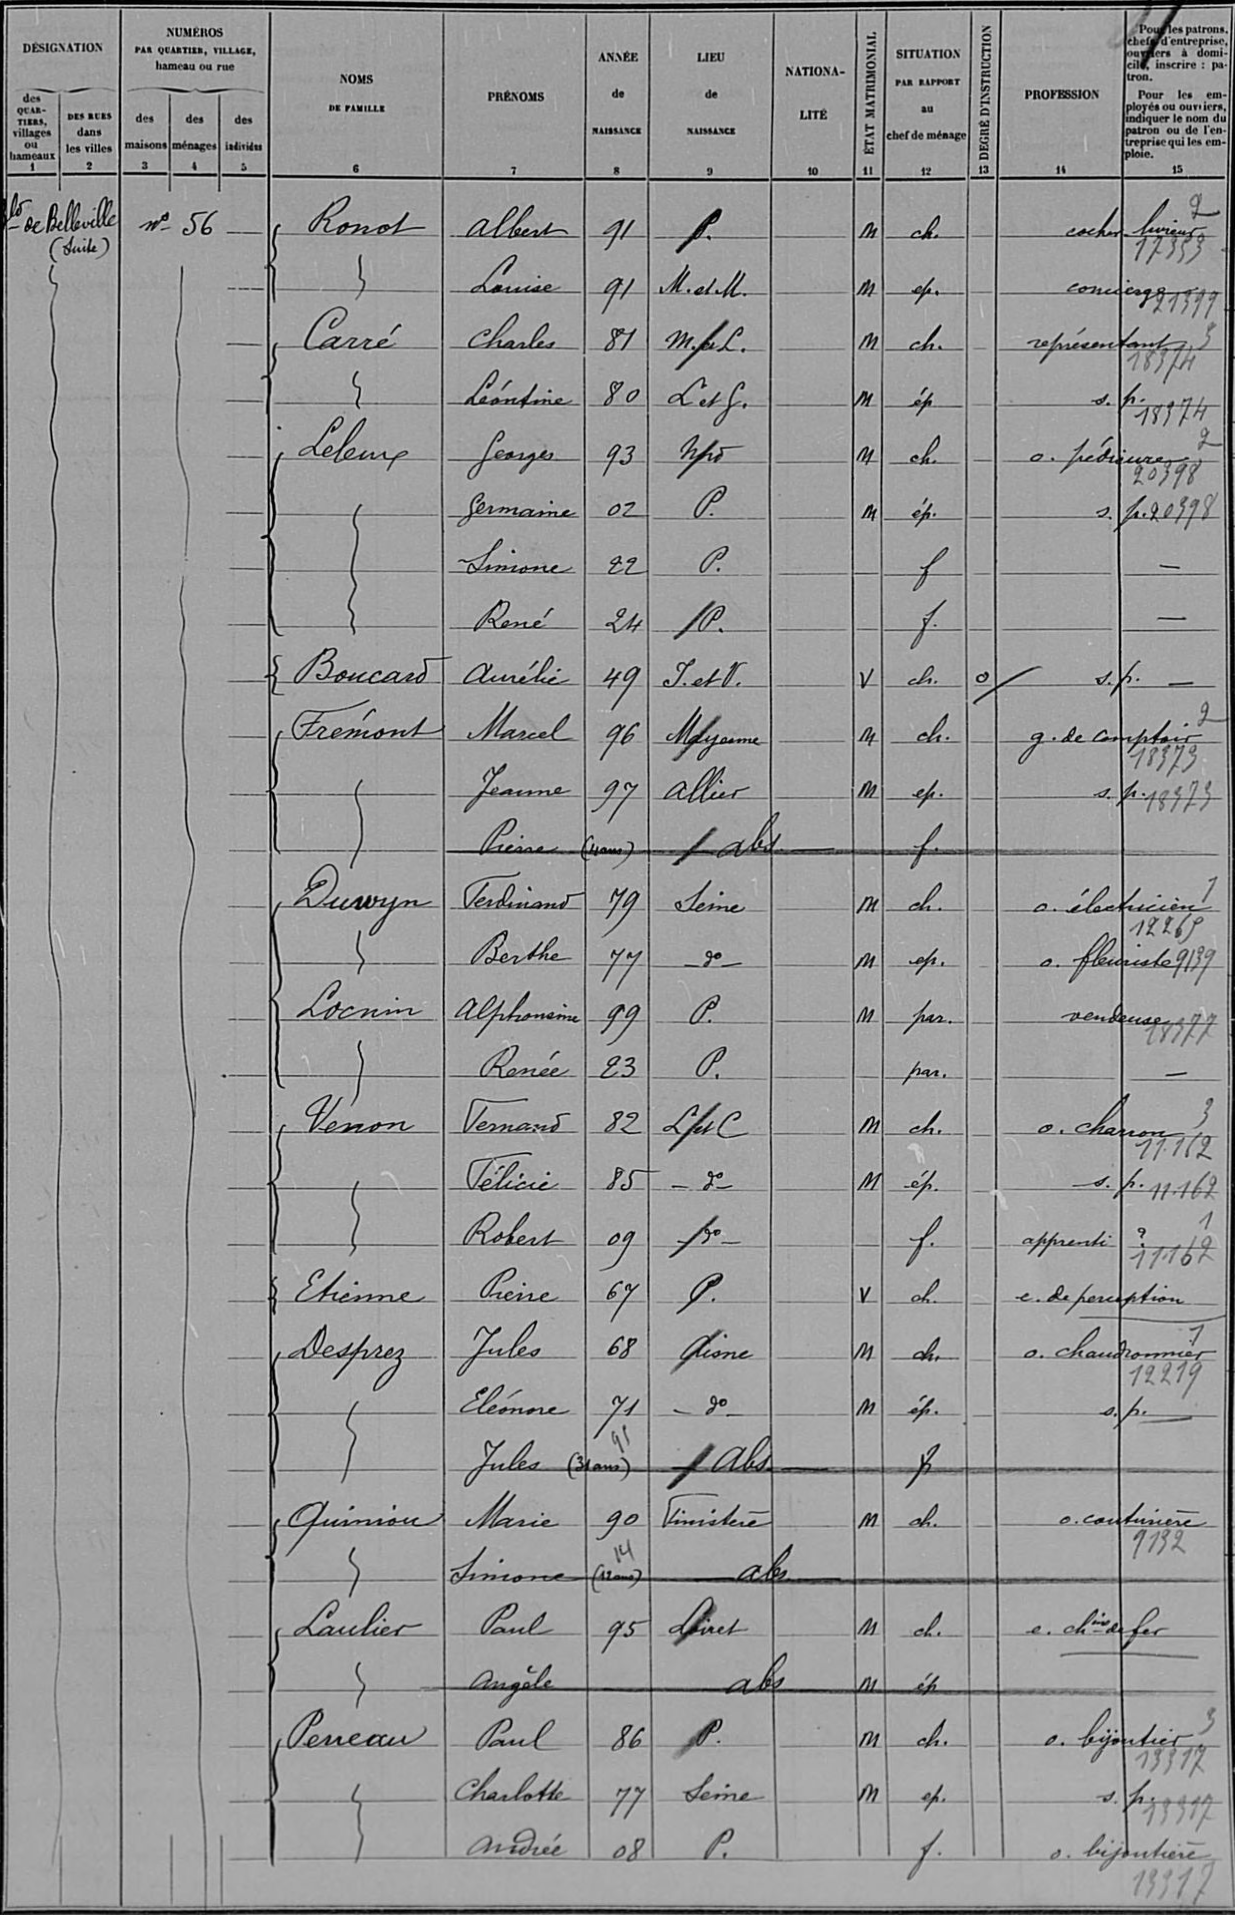Ronot/Albert/91/P /¤/M/ch /¤/cocher-livreur/¤
¤/Louise/91/M et M /¤/M/ep /¤/concierge/¤
Carré/Charles/81/M et L /¤/M/ch /¤/représentant/¤
¤/Léontine/80/L et G /¤/M/ép/¤/s p /18374
Leleux/Georges/93/Nord/¤/M/ch /¤/o pédicure/¤
¤/Germaine/02/P /¤/M/ép /¤/s p /20398
¤/Simone/22/P /¤/¤/f/¤/¤/¤/¤
¤/René/24/P /¤/¤/f /¤/¤/¤/¤
Boucard/Aurélie/49/I et V /¤/V/ch /o/s p /¤/¤
Frémont/Marcel/96/Mayenne/¤/M/ch /¤/g de comptoir/¤
¤/Jeanne/97/Allier/¤/M/ep /¤/s p /18373
¤/Pierre/(4 ans)/abs/¤/¤/f /¤/¤/¤/¤
Duwyn/Ferdinand/79/Seine/¤/M/ch /¤/o électricien/¤
¤/Berthe/77/d°/¤/M/ep /¤/o fleuriste/9139
Locnin/Alphonsine/99/P /¤/M/par /¤/vendeuse/¤
¤/Renée/23/P /¤/¤/par /¤/¤/¤/¤
Venon/Fernand/82/L et C/¤/M/ch /¤/o charron/11162 3
Félicie/85/d°/¤/M/ép /¤/s p /11162 1
¤/Robert/09/d°/¤/¤/f /¤/apprenti/¤
Etienne/Pierre/67/P /¤/V/ch /¤/e de perception/¤/¤
Desprez/Jules/68/Aisne/¤/M/ch /¤/o chaudronnier/¤
¤/Eléonore/71/d°/¤/M/ép /¤/s p /¤/¤
¤/Jules/(31 ans) 95/abs/¤/¤/f/¤/¤/¤
Quiniou/Marie/90/Finistère/¤/M/ch /¤/o couturière/¤
¤/Simone/(12 ans) 14/abs/¤/¤/¤/¤/¤/¤/¤
Laulier/Paul/95/Loiret/¤/M/ch /¤/e chin de fer/¤/¤
¤/Angèle/¤/abs/¤/M/ép/¤/¤/¤/¤
Perreau/Paul/86/P /¤/M/ch /¤/o bijoutier/¤
¤/Charlotte/77/Seine/¤/M/ep /¤/s p /13317
¤/Andrée/08/P /¤/¤/f /¤/o bijoutière/¤
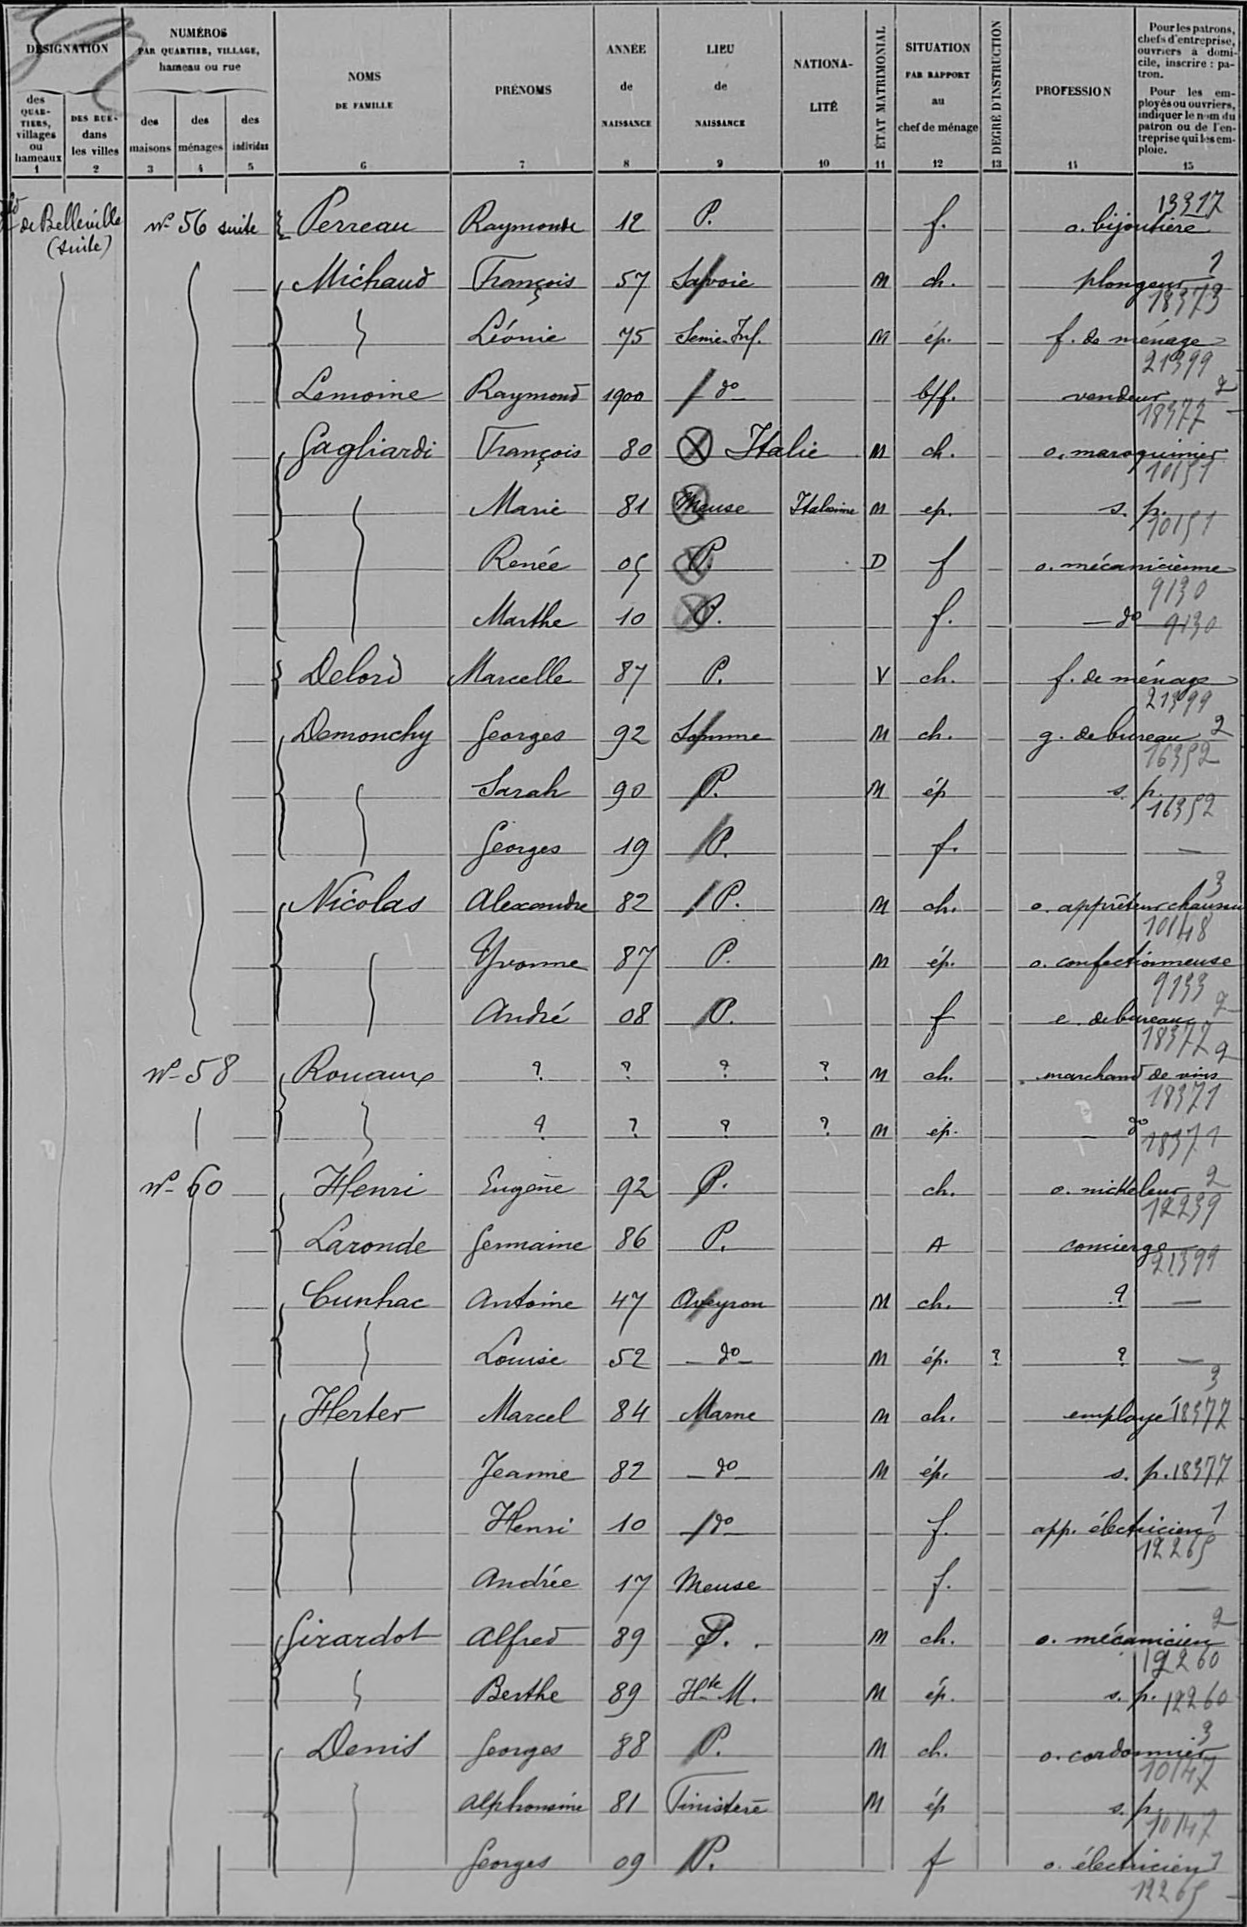Perreau/Raymonde/12/P /¤/¤/f /¤/o bijoutière/¤
Michaud/François/57/Savoie/¤/M/ch /¤/plongeur/¤
¤/Léonie/75/Seine Inf /¤/M/ép /¤/f de ménage/¤
Lemoine/Raymond/1900/d°/¤/¤/b-f /¤/vendeur/¤
Gagliardi/François/80/Italie/¤/M/ch /¤/o maroquinier/¤
¤/Marie/81/Meuse/Italienne/M/ep /¤/s p /¤
¤/Renée/05/P /¤/D/f/¤/o mécanicienne/¤
¤/Marthe/10/P /¤/¤/f /¤/d°/9130
Delord/Marcelle/87/P /¤/V/ch /¤/f de ménage/¤
Demonchy/Georges/92/Somme/¤/M/ch /¤/g de bureau/¤
¤/Sarah/90/P /¤/M/ép/¤/s p /¤
¤/Georges/19/P /¤/¤/f /¤/¤/¤/¤
Nicolas/Alexandre/82/P /¤/M/ch /¤/o apprêteur chausseur/¤
¤/Yvonne/87/P /¤/M/ép /¤/o confectionneuse/¤
¤/André/08/P /¤/¤/f/¤/e de bureaux/¤
Rouaux/¤/¤/¤/¤/M/ch /¤/marchand de vins/¤
¤/¤/¤/¤/¤/M:ép /¤/s°/18371
Henri/Eugène/92/P /¤/¤/ch /¤/o nickeleur/¤
Laronde/Germaine/86/P /¤/¤/A/¤/concierge/¤
Cunhac/Antoine/47/Aveyron/¤/M/ch /¤/¤/¤/¤
¤/Louise/52/d°/¤/M/ép /¤/¤/¤/¤
Herter/Marcel/84/Marne/¤/M/ch /¤/employé/18577
¤/Jeannie/82/d°/¤/M/ép /¤/s p /18377
¤/Henri/10/d°/¤/¤/f /¤/app électricien/¤
¤/Andrée/17/Meuse/¤/¤/f /¤/¤/¤
Girardot/Alfred/89/P /¤/M/ch /¤/o mécanicien/¤
¤/Berthe/89/Hte M /¤/M/ép /¤/s p /12260
Denis/Georges/88/P /¤/M/ch /¤/o cordonnier/¤
¤/Alphonsine/81/Finistère/¤/M/ép/¤/s p /10147
¤/Georges/09/P /¤/¤/f/¤/o electricien/¤
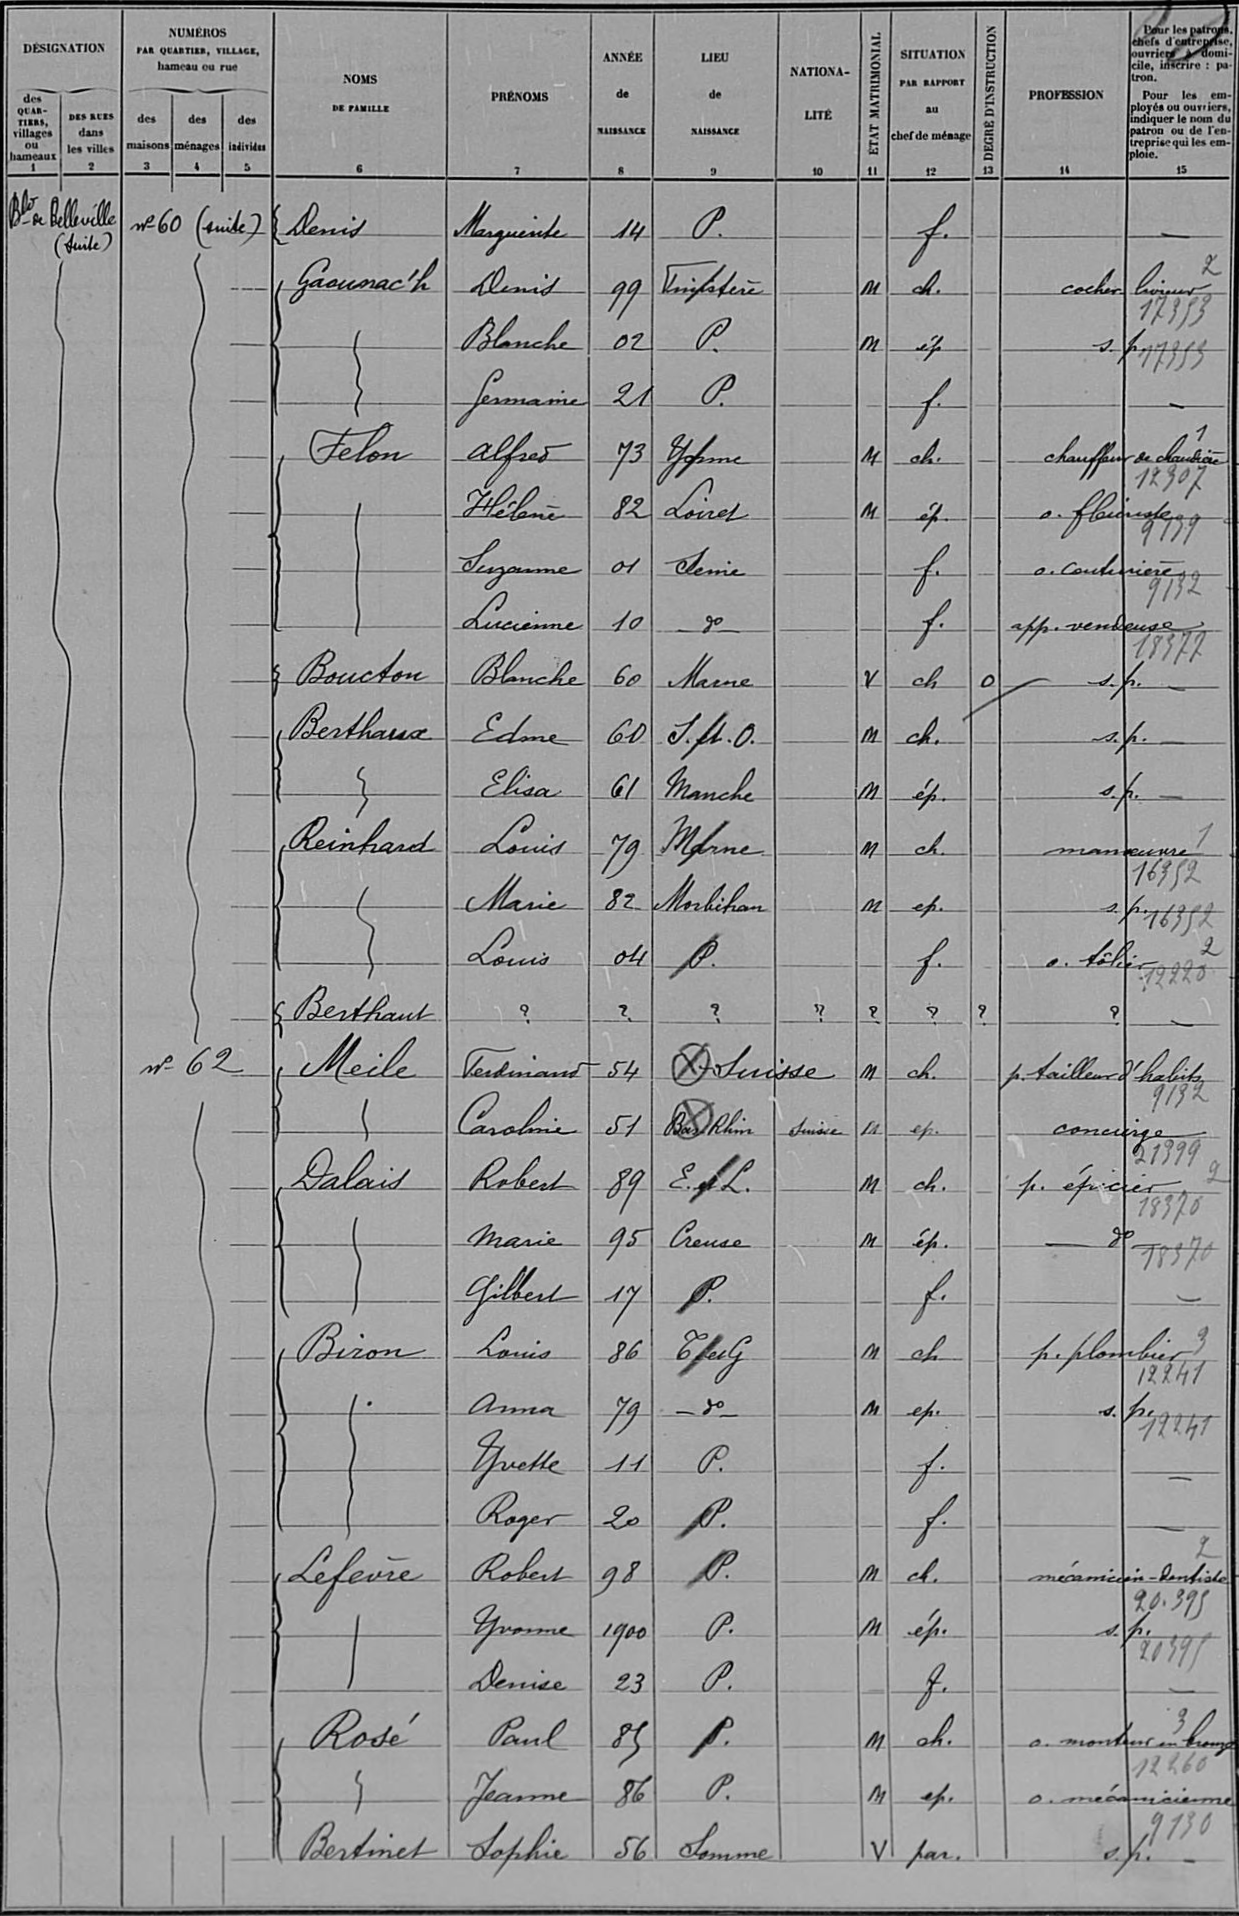Denis/Marguerite/14/P /¤/¤/f /¤/¤/¤/¤
Gaounac'h/Denis/99/Finistère/¤/M/ch /¤/cocher-livreur/¤
¤/Blanche/02/P /¤/M/ép/¤/s p /17353
¤/Germaine/21/P /¤/¤/f /¤/¤/¤/¤
Felon/Alfred/73/Yonne/¤/M/ch /¤/chauffeur de chaudière/¤
¤/Hélène/82/Loiret/¤/M/ép /¤/o fleuriste/¤
¤/Suzanne/01/Seine/¤/¤/f /¤/o couturière/¤
¤/Lucienne/10/d°/¤/¤/f /¤/app vendeuse/¤
Bouchon/Blanche/60/Marne/¤/V/ch/o/s p /¤/¤
Berthaux/Edme/60/S et O /¤/M/ch /¤/s p /¤/¤
¤/Elisa/61/Manche/¤/M/ép /¤/s p /¤/¤
Reinhard/Louis/79/Marne/¤/M/ch /¤/manoeuvre/¤
¤/Marie/82/Morbihan/¤/M/ep /¤/s p /16352
¤/Louis/04/P /¤/¤/f /¤/o tôlier/122202
Berthaut/¤/¤/¤/¤/¤/¤/¤/¤/¤/¤
Meile/Ferdinand/54/Suisse/¤/M/ch /¤/p tailleur d'habits/¤
¤/Caroline/51/Bas-Rhin/¤/Suisse/M/ép /¤/concierge/¤
Dalais/Robert/89/E et L /¤/M /ch /¤/p épicier/¤
¤/Marie/95/Creuse/¤/M/ép /¤/d°/¤
¤/Gilbert/17/P /¤/¤/f /¤/¤/¤/¤
Biron/Louis/86/T et G/¤/M/ch/¤/p plombier/¤
¤/anna/79/d°/¤/M/ep /¤/s p /¤
¤/Yvette/11/P /¤/¤/f /¤/¤/¤/¤
¤/Roger/20/P /¤/¤/f /¤/¤/¤
Lefèvre/Robert/98/P /¤/M/ch /¤/mécanicien-dentiste/¤
¤/Yvonne/1900/P /¤/M/ép /¤/s p /¤
¤/Denise/23/P /¤/¤/f /¤/¤/¤/¤
Rosé/Paul/85/P /¤/M/ch /¤/o monteur en bronze/¤
¤/Jeanne/86/P /¤/M/ep /¤/o mécanicienne/¤
Bertinet/Sophie/56/Somme/¤/V/par /¤/s p /¤/¤